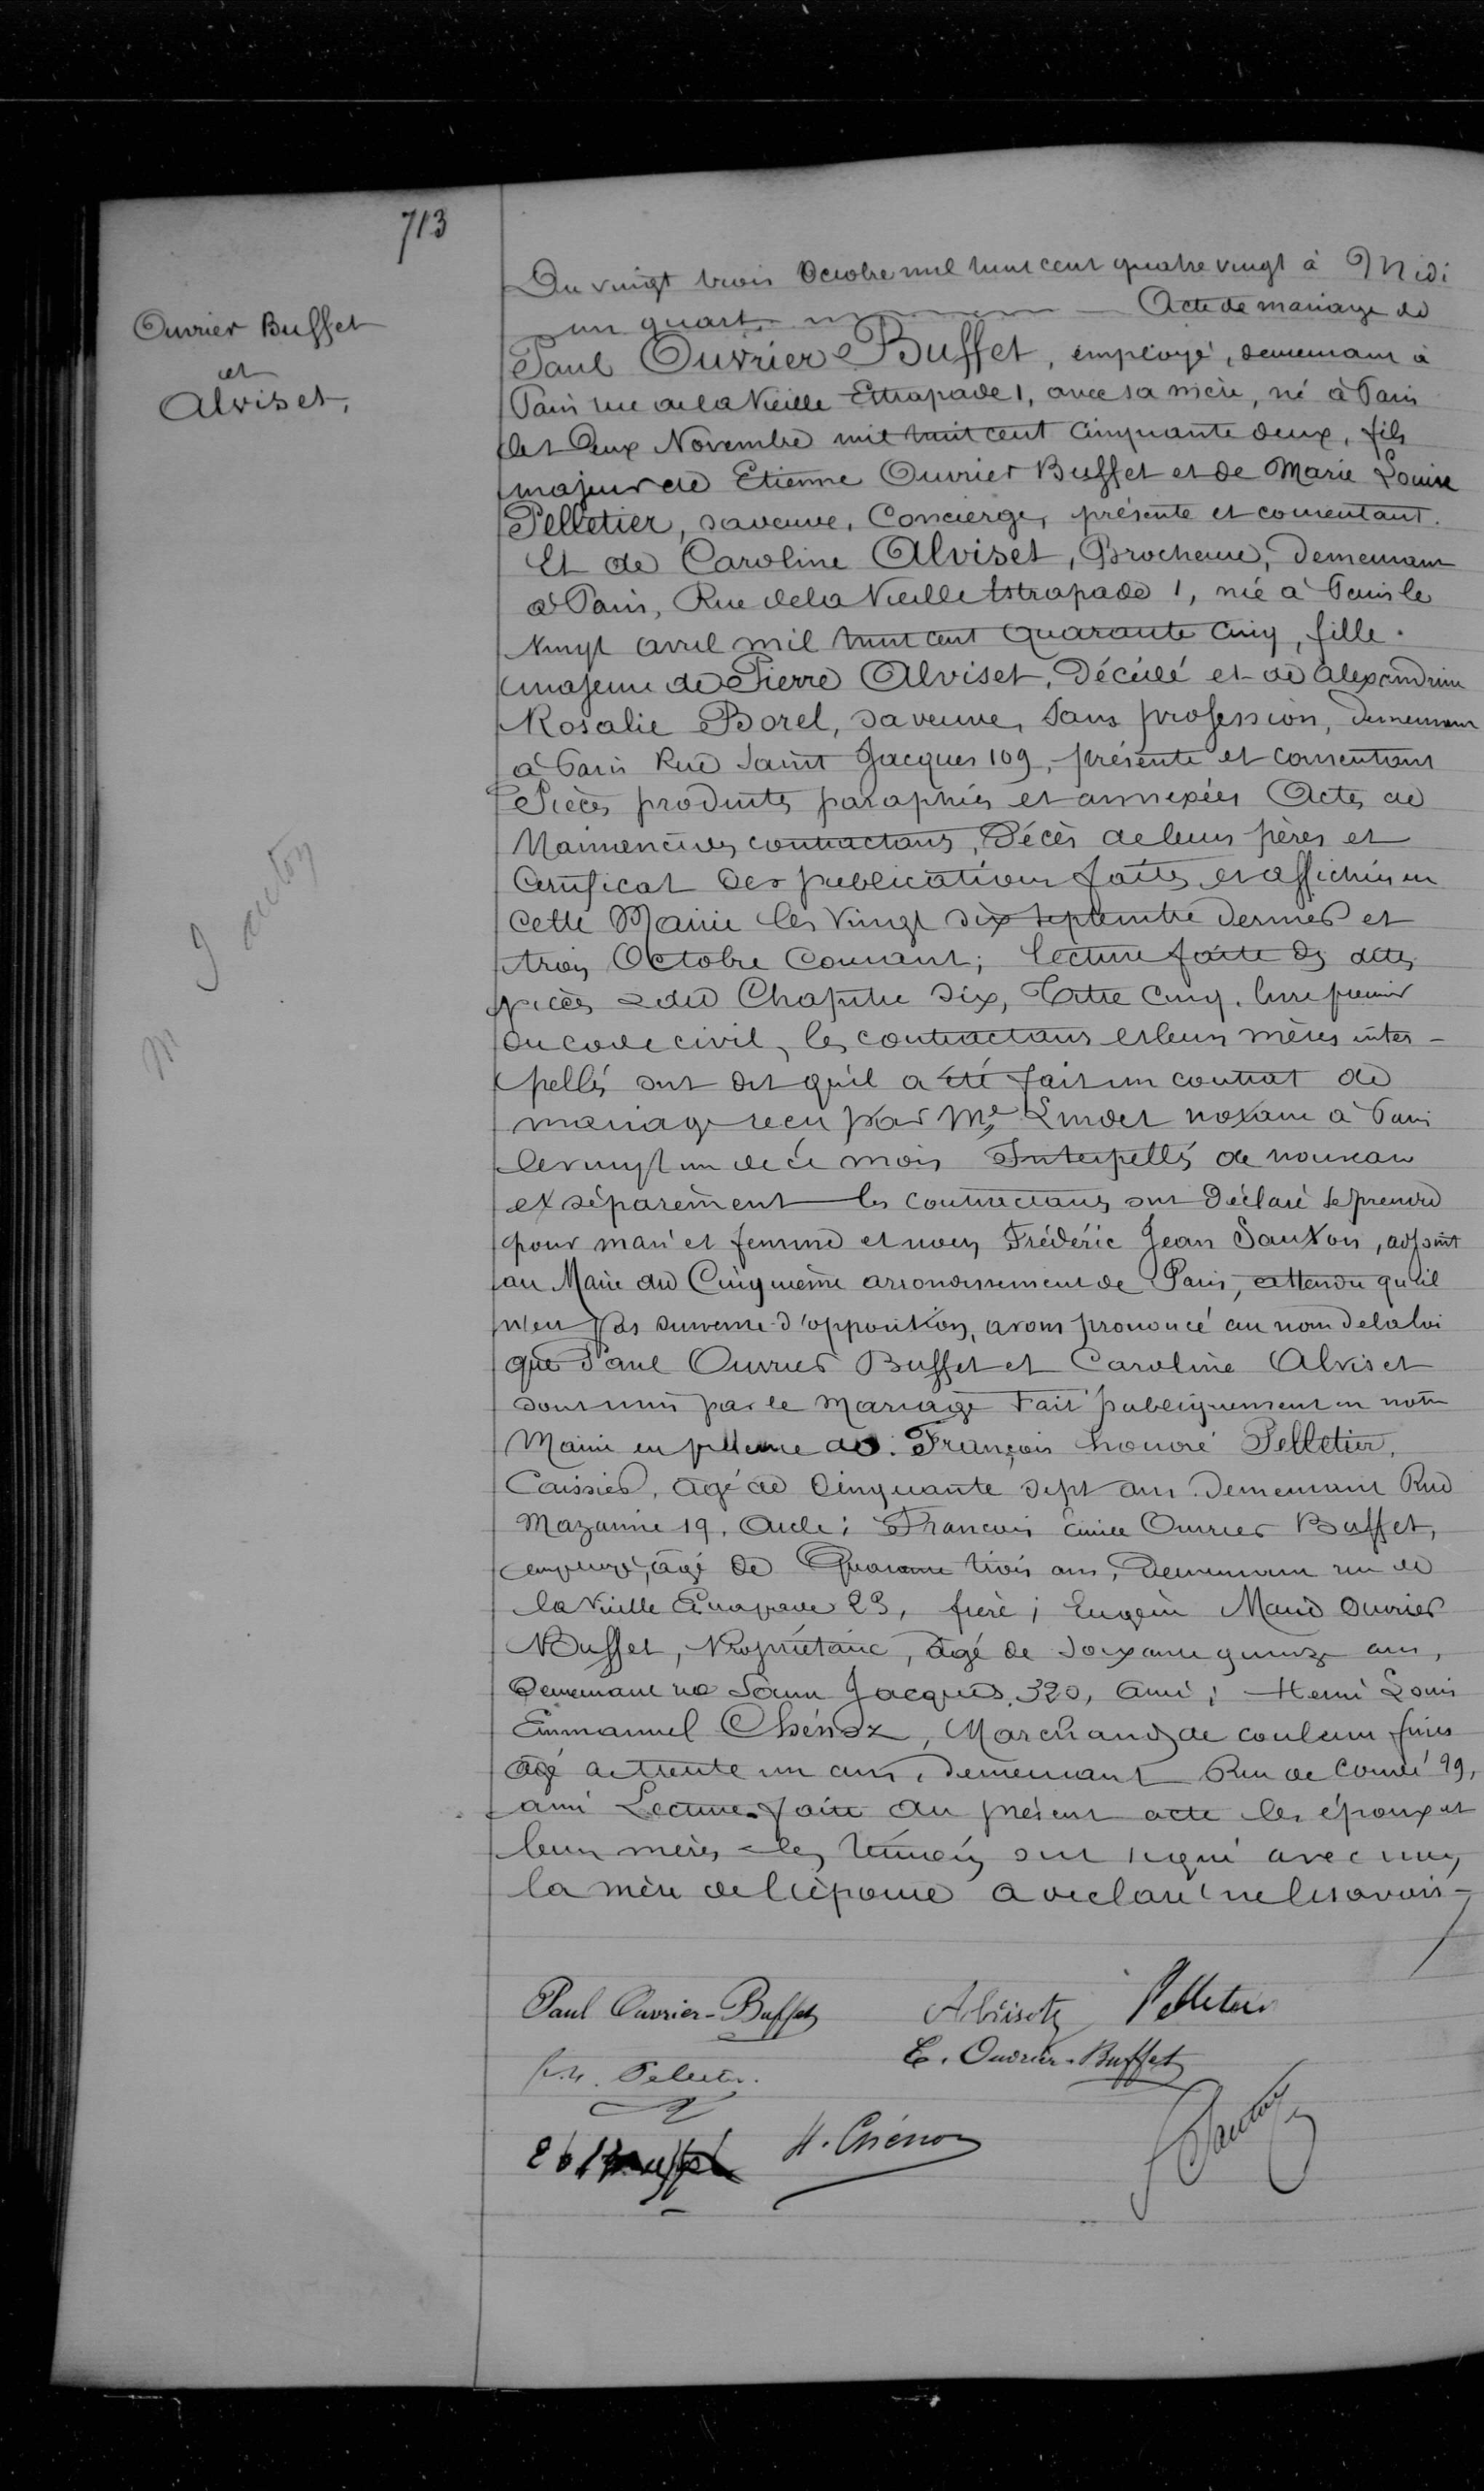713
Ouvrier Buffet
et
Alviset
Du vingt trois Octobre mil huit cent quatre vingt à Midi
un quart. Acte de mariage de
Paul Ouvrier Buffet, employé, demeurant à
Paris rue de la Vieille Estrapade 1, avec sa mère, né à Paris
le deux Novembre mil huit cent cinquante deux, fils
majeur de Etienne ouvrier Buffet et de Marie Louise
Pelletier, sa veuve, concierge, présente et consentant
Et de Caroline Alviset, Brocheuse demeurant
à Paris, Rue de la Vieille Estrapade, née à Paris le
vingt avril mil huit cent quarante cinq, fille
majeure de Pierre Alviset, décédé et de Alexandrine
Rosalie Boret, sa veuve, sans profession, demeurant
à Paris Rue Saint Jacques 109, présente et consentant
Pièces produites paraphées et annexées Acte de
naissance des contractants, Décès de leurs pères et
Certificat des publication faites et affichées en
cette Mairie les vingt six septembre dernier et
trois Octobre courant; lecture faite les dites
pièces du Chapitre six, Titre cinq, livre premier
du code civil, les contractants et leur mères inter-
pellés ont dit qu'il a été fait un contrat de
mariage reçu par Me Linder notaire à Paris
le vingt un de ce mois Interpellés de nouveau
et séparément les contractants ont déclaré se prendre
pour mari et femme et nous Frédéric Jean Sauton, adjoint
au Maire du Cinquième arrondissement de Paris, attendre qu'il
n'eu pas survenu d'opposition, avons prononcé au nom de la loi
que Paul Ouvrier Buffet et Caroline Alviset
sont unis par le mariage fait publiquement en notre
Mairie en présence de: François Honoré Pelletier,
Caissier, âgé de cinquante sept ans, demeurant Rue
Mazarine 19, Oncle: François Emile Ourrier Buffet,
employé, âgé de quarante trois ans, demeurant rue de
la Vieille Estrapade 23, frère; Eugène Marie Ourrier
Buffet, propriétaire, âgé de soixante quinze ans,
demeurant rue Saint Jacques 320, ami: Henri Louis
Emmanuel Chenoz, Marchand de couleur frères
Agé de trente un an, demeurant Rue de Condé 29
ami Lecture faite au présent acte les époux et
leur mères, les témoins ont signé avec nous,
la mère de l'époux a déclaré ne les avoir
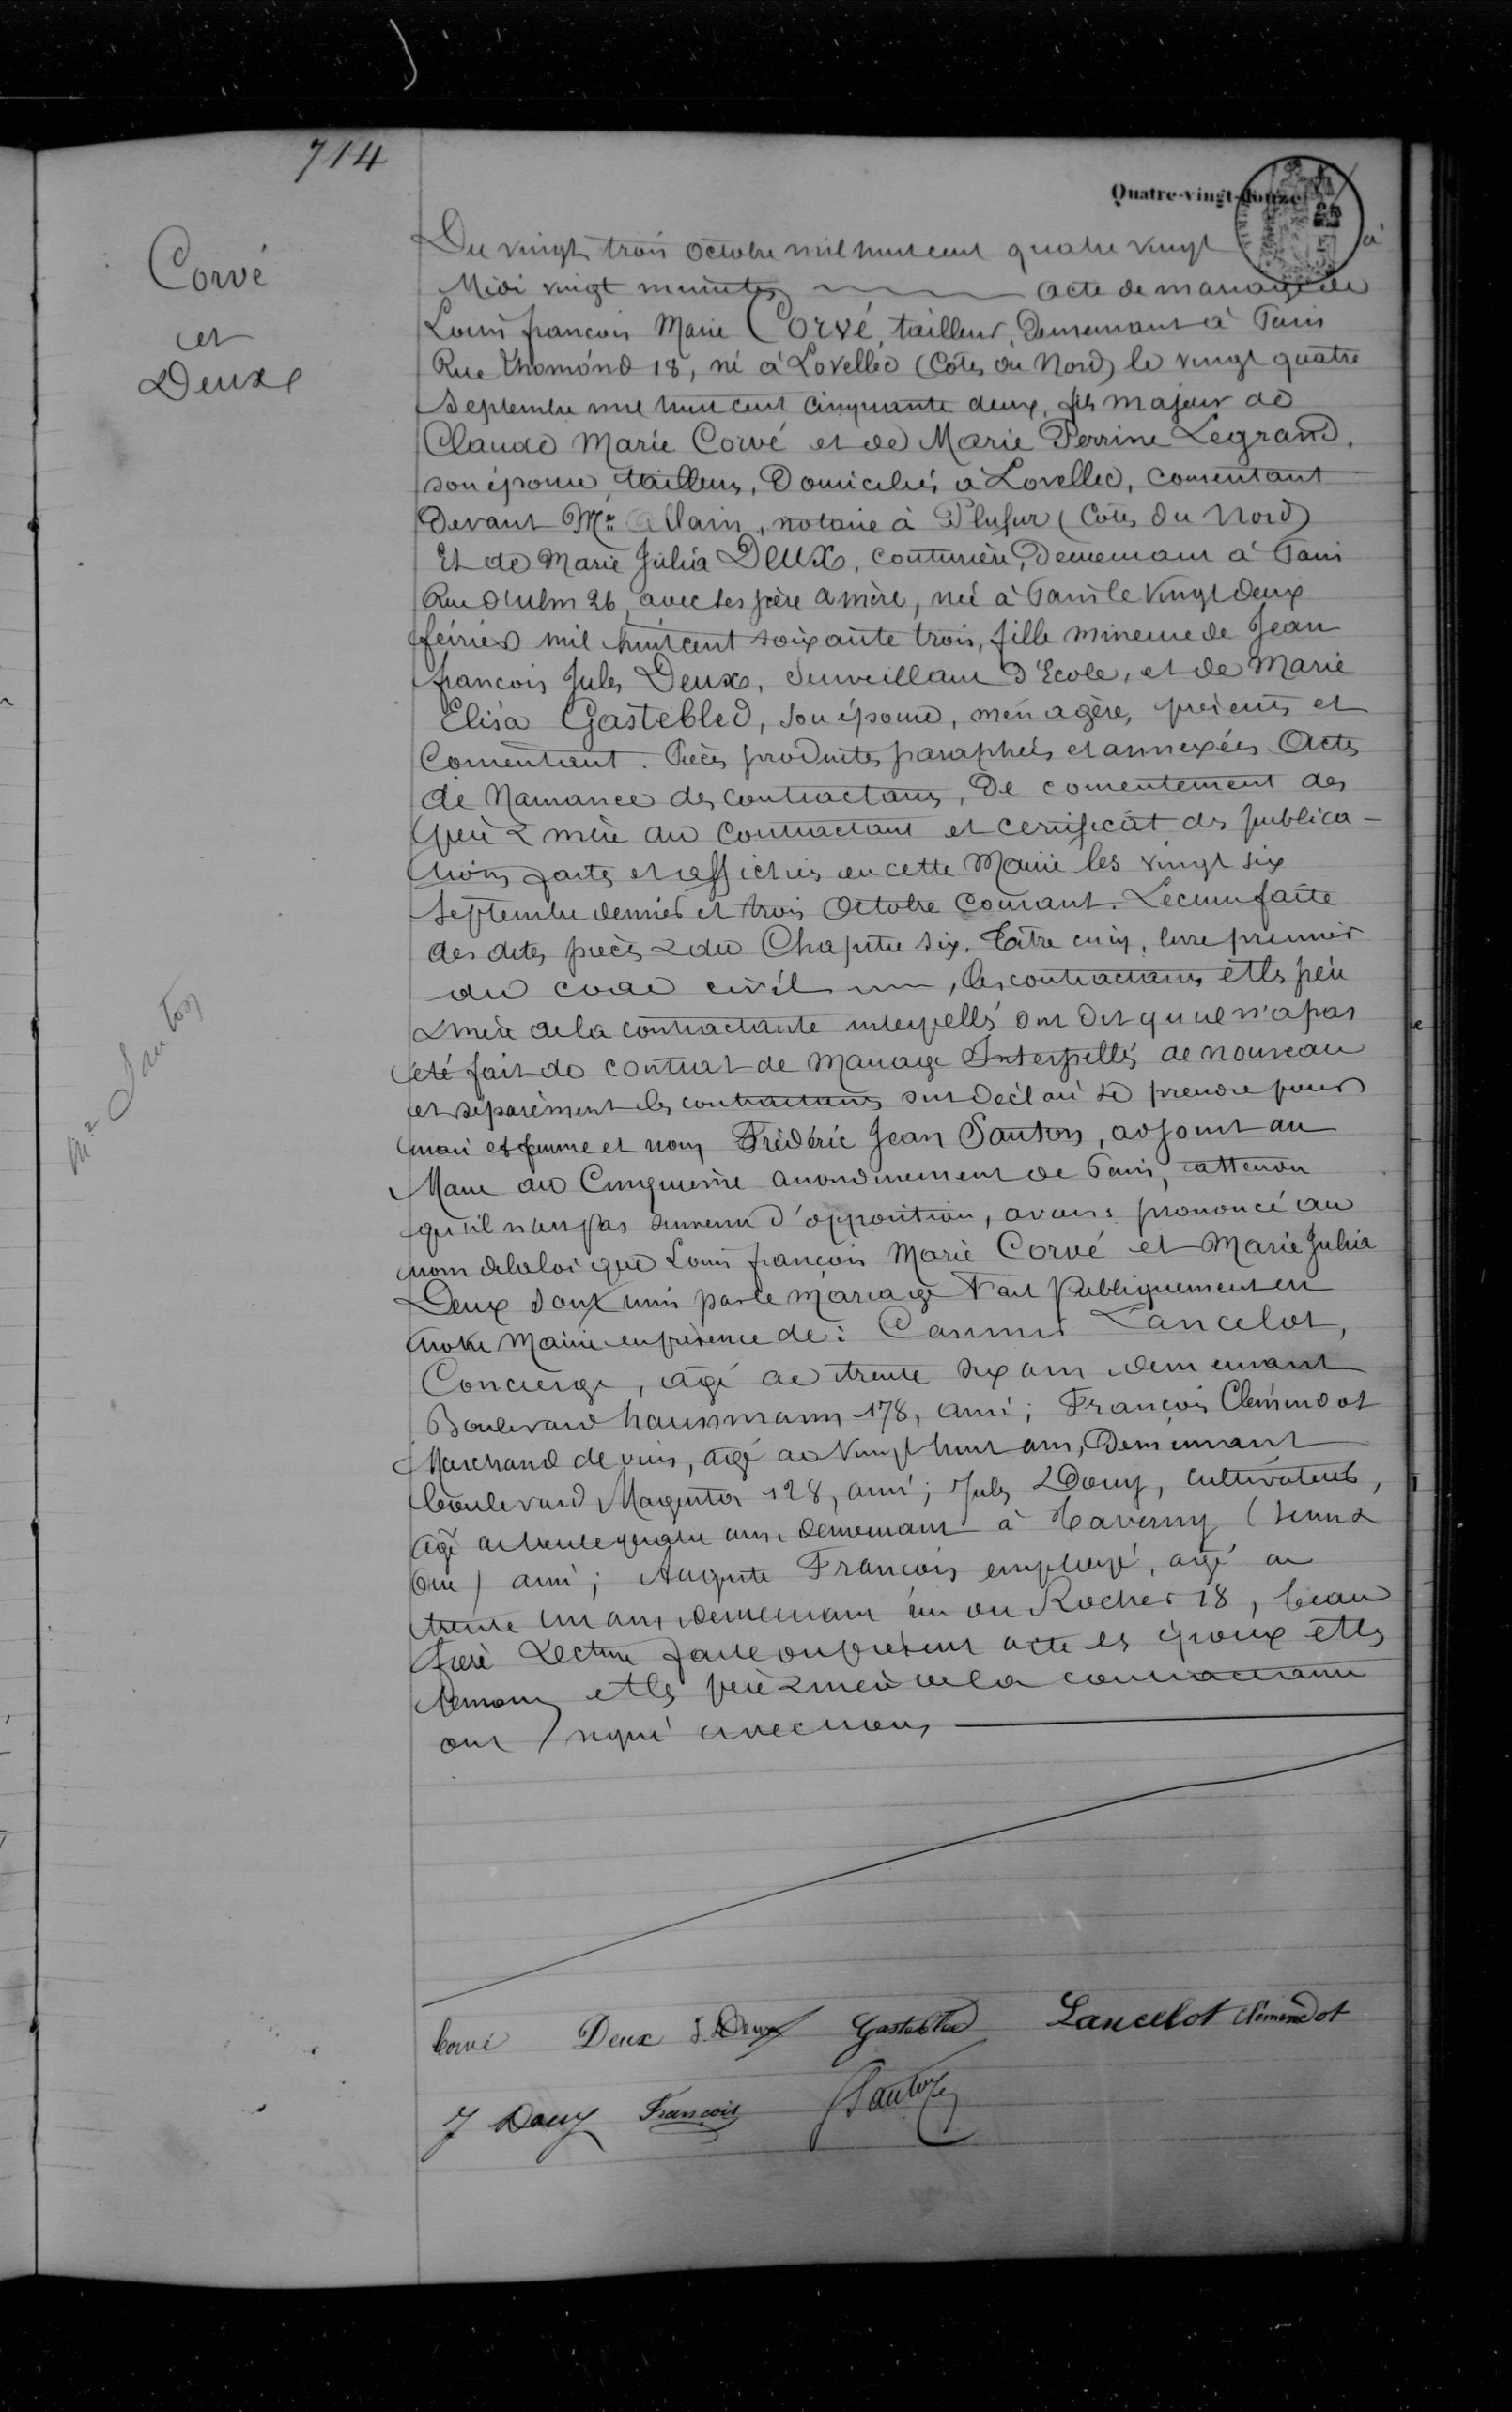714
Corvé
et
Deux
Le vingt trois Octobre mil neuf cent quatre vingt
midi vingt minutes -Acte de mariage de
Louis François Marie Corvé, tailleur, demeurant à Paris
Rue Thomond 18, né à Lovellec (Côtes du Nord) le vingt quatre
Septembre mil neuf cent cinquante deux, fils majeur de
Claude Marie Corvé et de Marie Perrine Legrand,
son épouse, tailleur, domiciliés à Lovellec, consentant
Devant Me Allain, notaire à Plufur (Côtes du Nord)
Et de Marie Julia Deux, couturière, demeurant à Paris
Rue d'Ulm 26, avec ses père & mère, née à Paris le vingt deux
février mil huit cent soixante trois, fille mineure de Jean
François Jules Deux, surveillant d'Ecole, et de Marie
Elisa Gastebled, son épouse, ménagère, présents et
Consentants. Pièces produites, paraphées et annexées. Actes
de naissance des contractants, de consentement des
père et mère au Contractant et certificat de publica-
tions faites et affichées en cette Mairie les vingt six
septembre dernier et trois Octobre courant. Lecture faite
des dites pièces & du Chapitre six, Titre cinq, livre premier
du code civil -,les contractants et les père
et mère de la contractante interpellés ont dit qu'il n'a pas
été fait de contrat de mariage Interpellés de nouveau
et séparément les contractants ont déclaré se prendre pour
mari et femme et nous Frédéric Jean Sanson, adjoint au
Maire du Cinquième arrondissement de Paris, attendu
qu'il n'eu pas survenu d'opposition, avons prononcé au
nom de la loi que Louis François Marie Corvé et Marie Julia
Deux sont unis par le mariage Fait publiquement en
notre Mairie en présence de: Casimir Lancelot,
Concierge, âge de trente six ans, demeurant
Boulevard Haussmann 178, ami; François Clémencot
Marchand de vins, âgé de vingt trois ans, demeurant
boulevard Magenta 128, ami; Jules Lecouy, cultivateur,
Agé de trente quatre ans, demeurant à Maverny, (Seine &
Oise) ami; Auguste François employé agé de
trente un an, demeurant rue du Rocher 18, beau
frère Lecture faite du présent acte les époux et les
témoins et les père & mère de la contractante
ont signé avec nous.